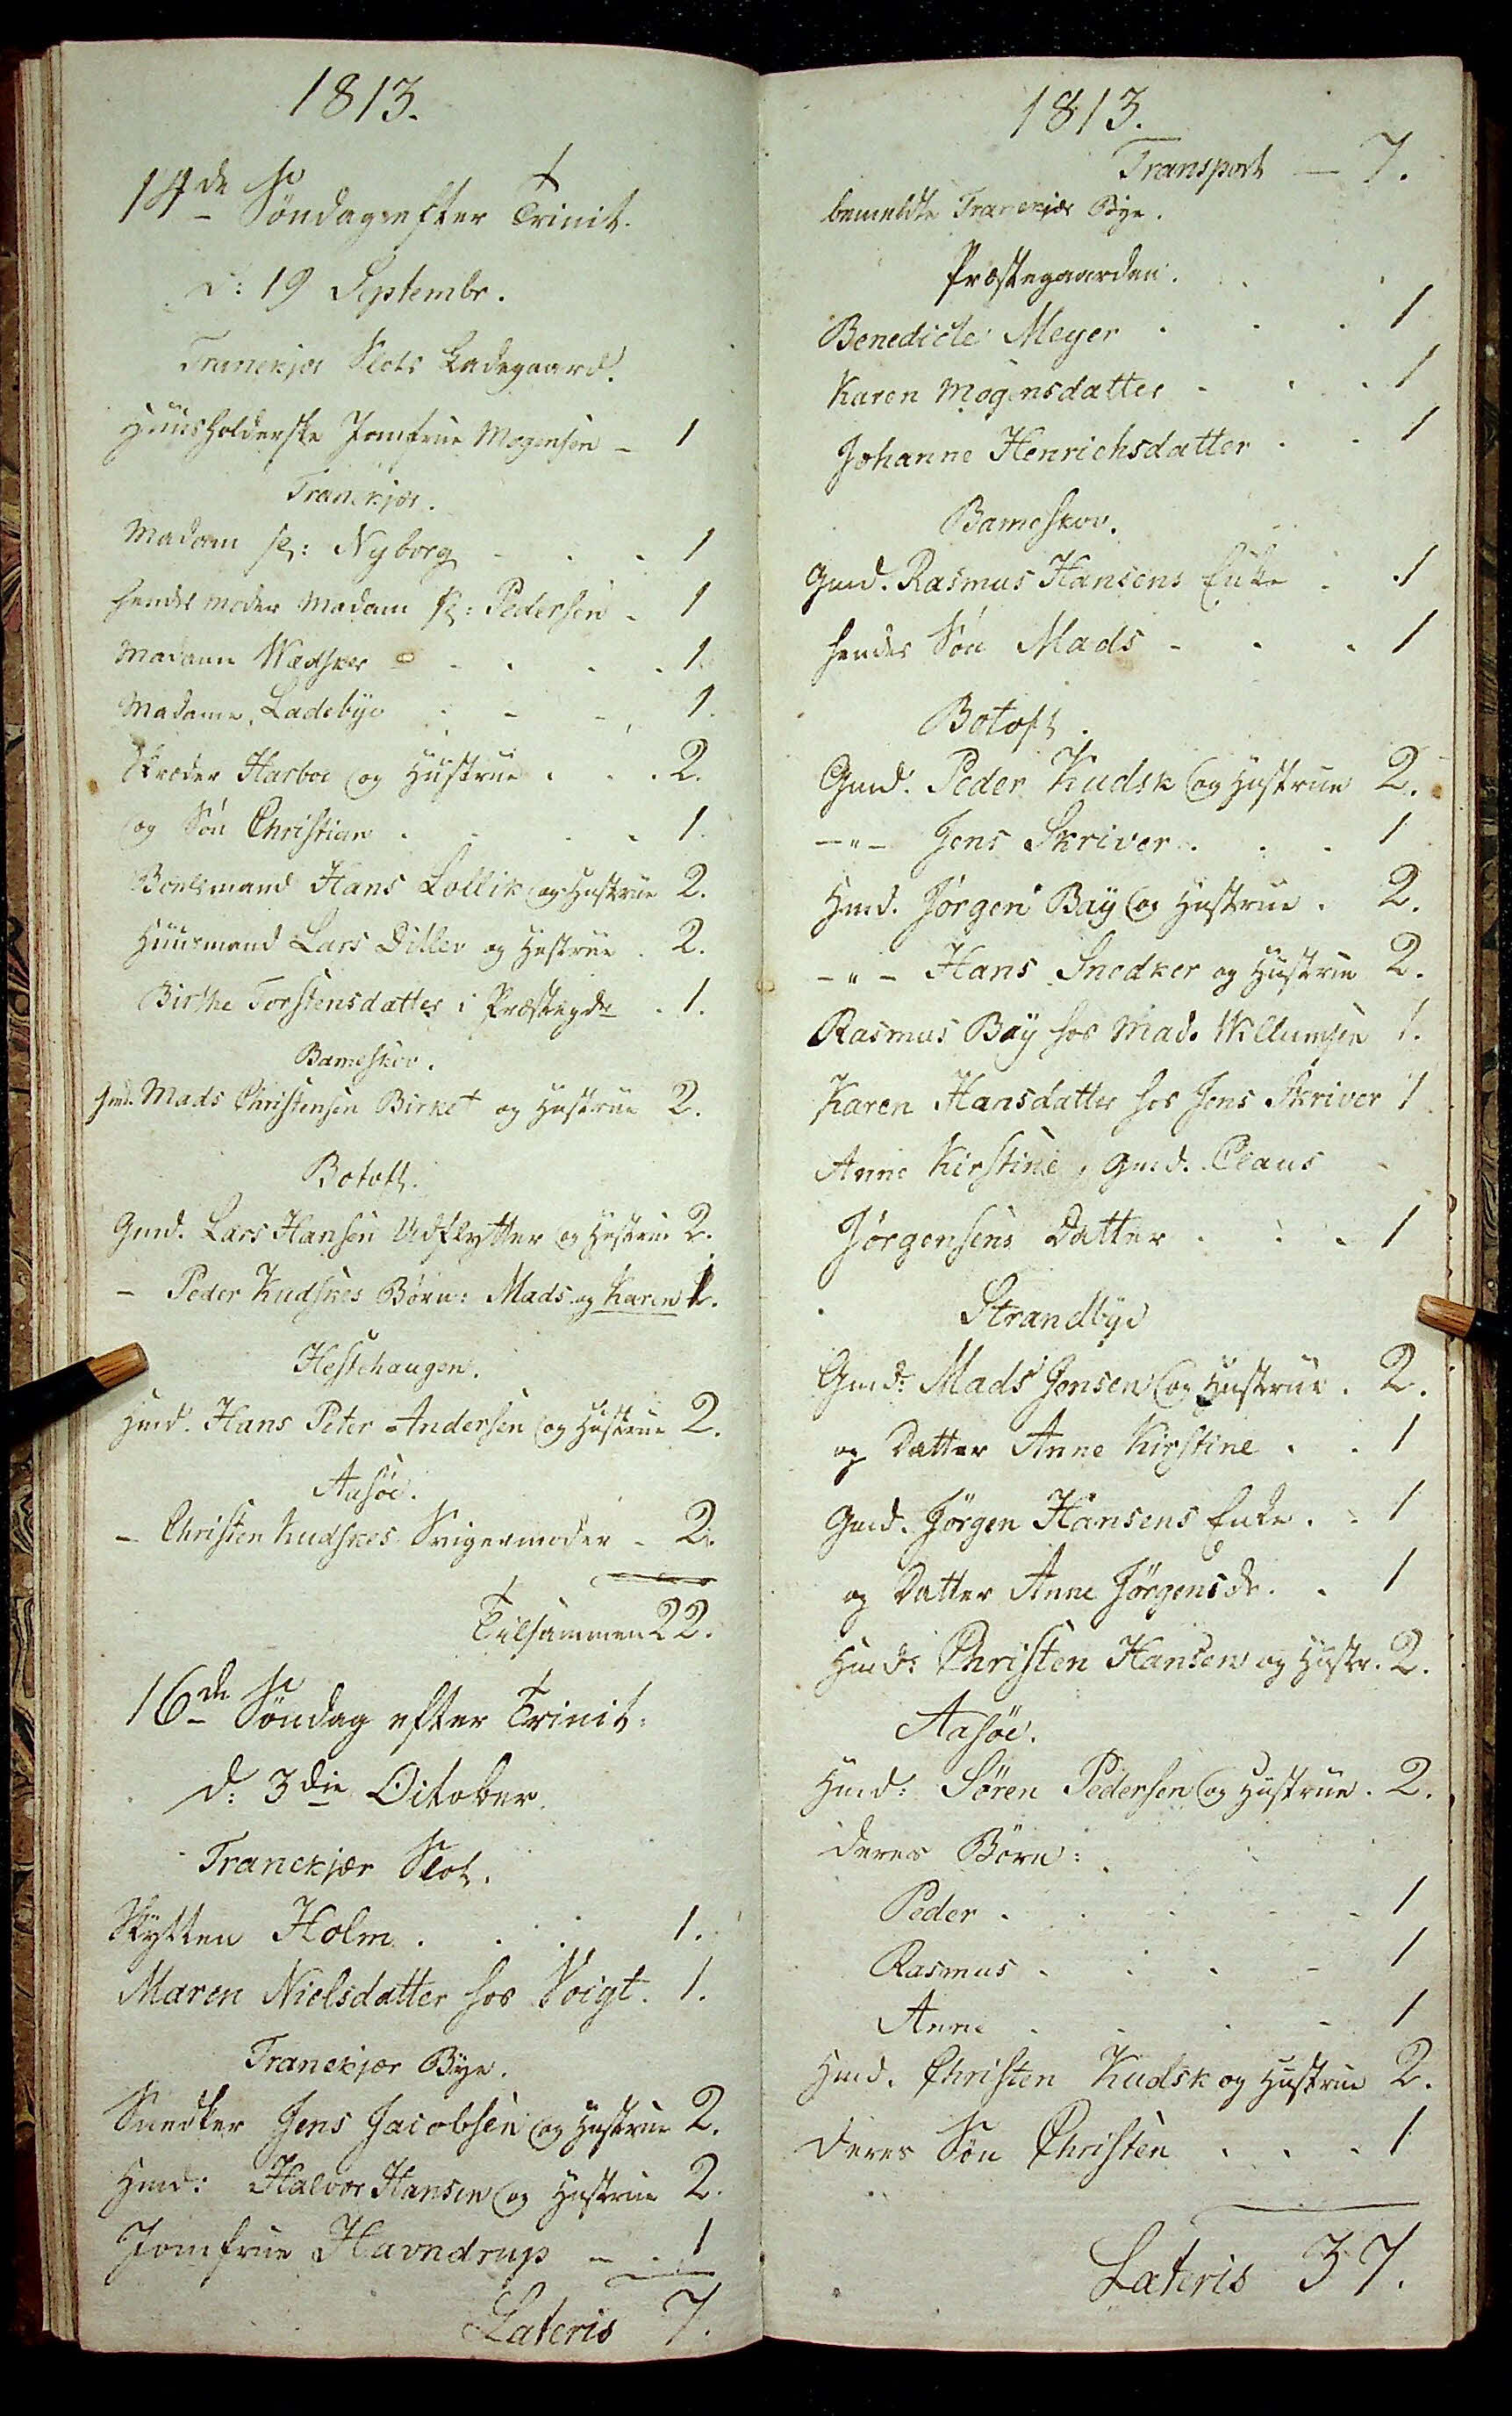1813.
14de Søndag efter Trinit.
d: 19 Septembr:
Tranekjær Slots Ladegaard.
Huusholderske Jomfrue Mogensen - 1
Tranekjær.
Madam sl: Nyborg . . . 1
hendes Moder Madam sl: Pedersen . 1
Madame Wædsker . . . . 1.
Madame Ladebye . . . , 1.
Skræder Harboe og Hustrue . . . 2.
og Søn Christian . . . . 1.
Boelsmand Hans Lollik og Hustrue 2.
Huusmand Lars Ditlev og Hustrue . 2.
Birthe Torstensdatter i Præstegdr . 1.
Bameskov.
Gmd. Mads Christensen Birket og Hustrue 2.
Botoft.
Gmd. Lars Hansen Udflytter og Hustrue 2.
- Peder Knudsøns Børn: Mads og Karen 1.
Hestehaugen.
Hmd. Hans Peter Andersen og Hustrue 2.
Aasøe.
- Christen Kudskes Svigermoder - 2.
Tilsammen 22.
16de Søndag efter Trinit:
d: 3die October.
Tranekjær Slot.
Skytten Holm . . . 1.
Maren Nielsdatter hos Voigt. 1.
Tranekjær Bye.
Snedker Jens Jacobsen og Hustrue 2.
Hmd: Halvor Hansen og Hustrue 2
Jomfrue Havndrup - . 1
Lateris 7.
1813.
Transport - 7.
bemeldte Tranekjær Bye.
Præstegaarden.
Benedicte Meyer . . . 1
Karen Mogensdatter . . . 1
Johanne Henrichsdatter . . 1
Bameskov.
Gmd. Rasmus Hansens Enke . . 1
hendes Søn Mads . . . 1
Botoft
Gmd. Peder Kudsk og Hustrue 2.
-"- Jens Skriver . . . 1
Hmd. Jørgen Bay og Hustrue . 2.
-"- Hans Snedker og Hustru 2.
Rasmus Bay hos Mads Willumsen 1.
Karen Hansdatter hos Jens Skriver 1
Anne Kirstine, Gmd. Claus
Jørgensens Datter . . . 1
Strandbye
Gund: Mads Jensens søn Hustrue. 2
og Datter Anne Kirstine . . 1
Gmd. Jørgen Hansens Enke . . 1
og Datter Anne Jørgensdr . . 1
Hand: Christen Hansen og Hustr . 2.
Aasøe.
Hmd. Søren Pedersen og Hustrue . 2.
deres Børn:
Peder . . . . . 1
Rasmus . . . . 1
Anne . . . . 1
Hmd. Christen Kudsk og Hustrue 2.
deres Søn Christen . . . 1
Lateris 37.
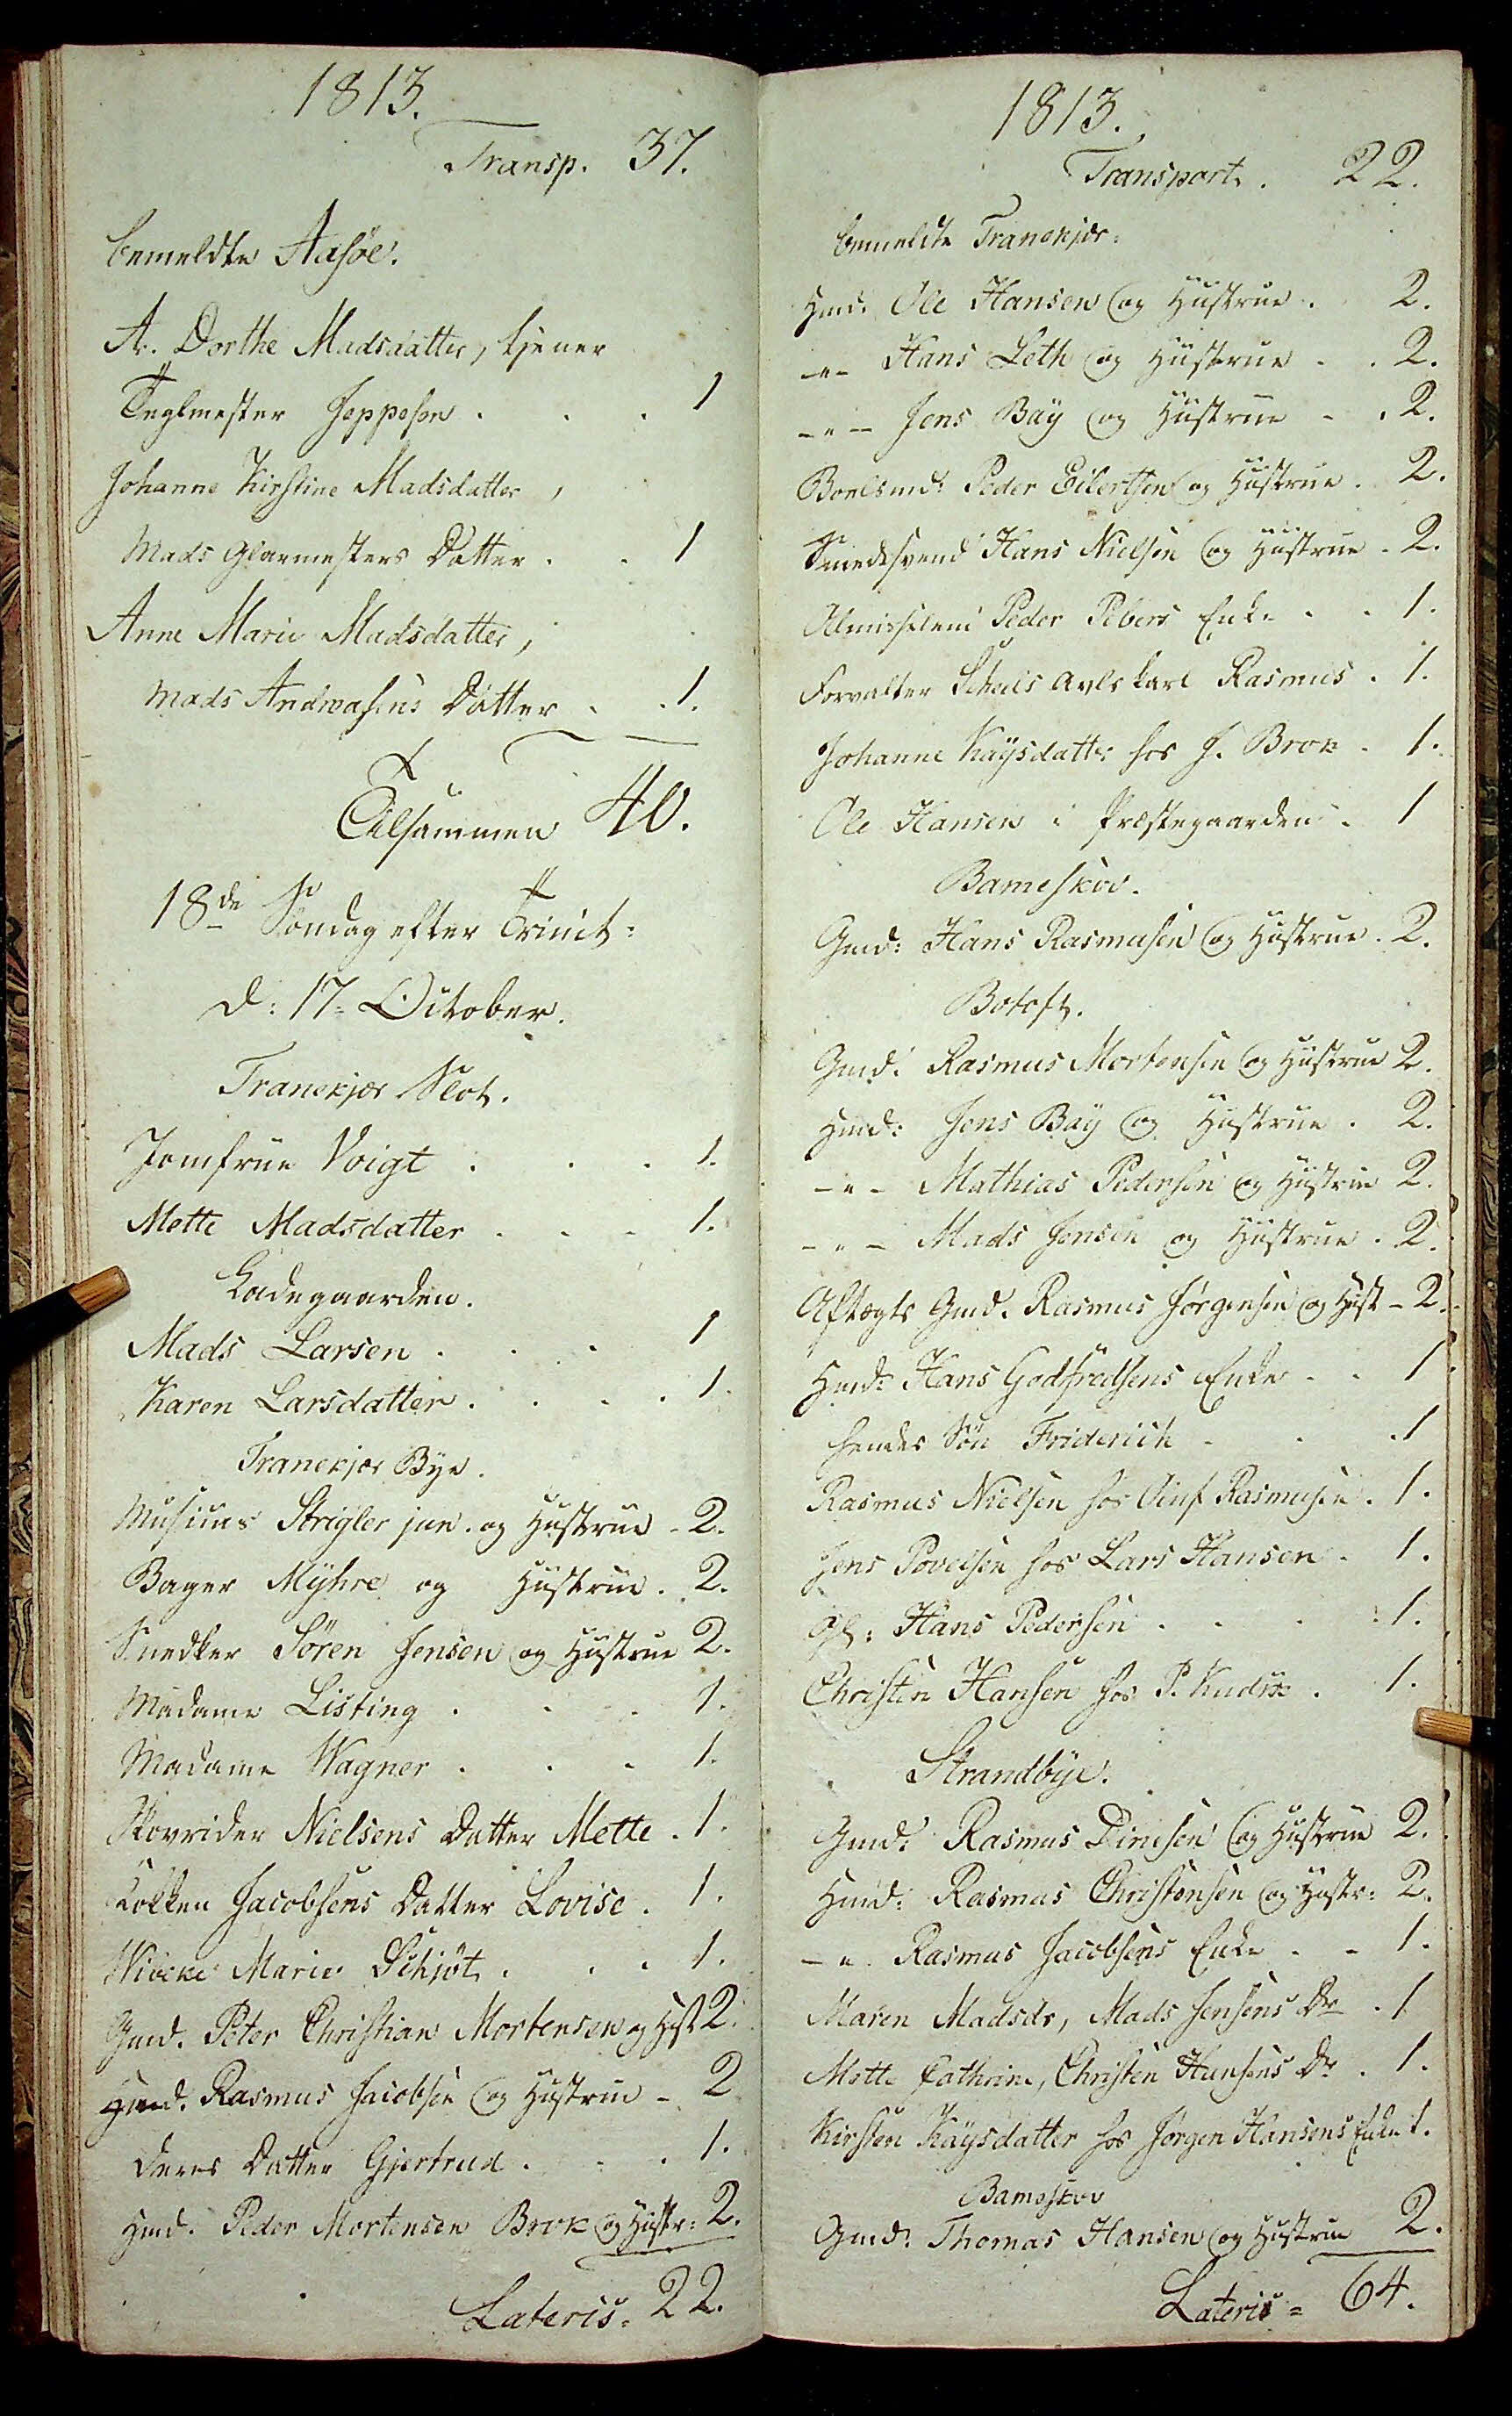1813.
Transp. 37.
bemeldte Aasøe.
A. Dorthe Madsdatter, tjener
Teglmester Jeppesen . . . 1
Johanne Kirstine Madsdatter
Mads Glarmesters Datter . . 1
Anne Maria Madsdatter
Mads Andreasens Datter . . 1.
Tilsammen 40.
18de Søndag efter Trinit:
d: 17. October.
Tranekjær Slot.
Jomfrue Voigt . . . 1.
Mette Madsdatter . . . 1.
Ladegaarden.
Mads Larsen . . . 1
Karen Larsdatter . . . . 1.
Tranekjær Bye.
Musicus Stringler jun. og Hustrue - 2.
Bager Myhre og Hustrue . 2.
Snedker Søren Jensen og Hustrue 2.
Madame Listing . . . 1.
Madame Wagner . . . 1.
Skovrider Nielsens Datter Mette . 1.
Kocken Jacobsens Datter Lovise . 1.
Wibeke Marie Schjøt . . . 1.
Gmd. Peter Christian Mortensen og Hst 2.
Hmd. Rasmus Jacobsen og Hustrue - 2
deres Datter Gjertrud . . . 1.
Hmd. Peder Mortensen Brok og Hustr : 2
Lateris. 22.
1813.
Transport. 22.
bemeldte Tranekjær.
Hmd. Ole Hansens og Hustrue . 2.
-"- Hans Leth og Hustrue . . 2.
-"- Jens Bay og Hustrue . .  2.
Boelsmd: Peder Eilertsen og Hustrue . 2.
Smedsvend Hans Nielsen og Hustrue - 2.
Almisselem Peder Pebers Enke . . 1.
Forvalter Scheels Avlskarl Rasmus. 1.
Johanne Kaysdatter hos J. Brok . 1.
Ole Hansen i Præstegaarden . 1
Bameskov.
Gmd: Hans Rasmusen og Hustrue . 2.
Botoft.
Gmd. Rasmus Mortensen og Hustrue 2.
Hmd: Jens Bay og Hustrue . 2.
-"- Mathias Pedersen og Hustrue 2.
-"- Mads Jensen og Hustrue . 2.
Aftægts Gmd. Rasmus Jørgensen og Hust - 2.
Hmd: Hans Godfredsens Enke . . 1
hendes Søn Friderich . . . 1
Rasmus Nielsen hos Jens Rasmusen . 1.
Jens Povelsen hos Lars Hansen . 1.
Gl: Hans Pedersen . . . . 1.
Christen Hansen hos P. Kunsk . 1.
Strandbye.
Gmd. Rasmus Dinesen og Hustrue 2.
Hmd: Rasmus Christensen og Hustr : 2.
-"- Rasmus Jacobsens Enke - - 1.
Maren Madsdr, Mads Jensens Dr . 1
Mette Cathrine, Christen Hansens Dr . 1.
Kirsten Kaysdatter hos Jørgen Hansens Enke 1.
Bammeskov
Gmd. Thomas Hansen og Hustrue 2.
Lateris = 64.